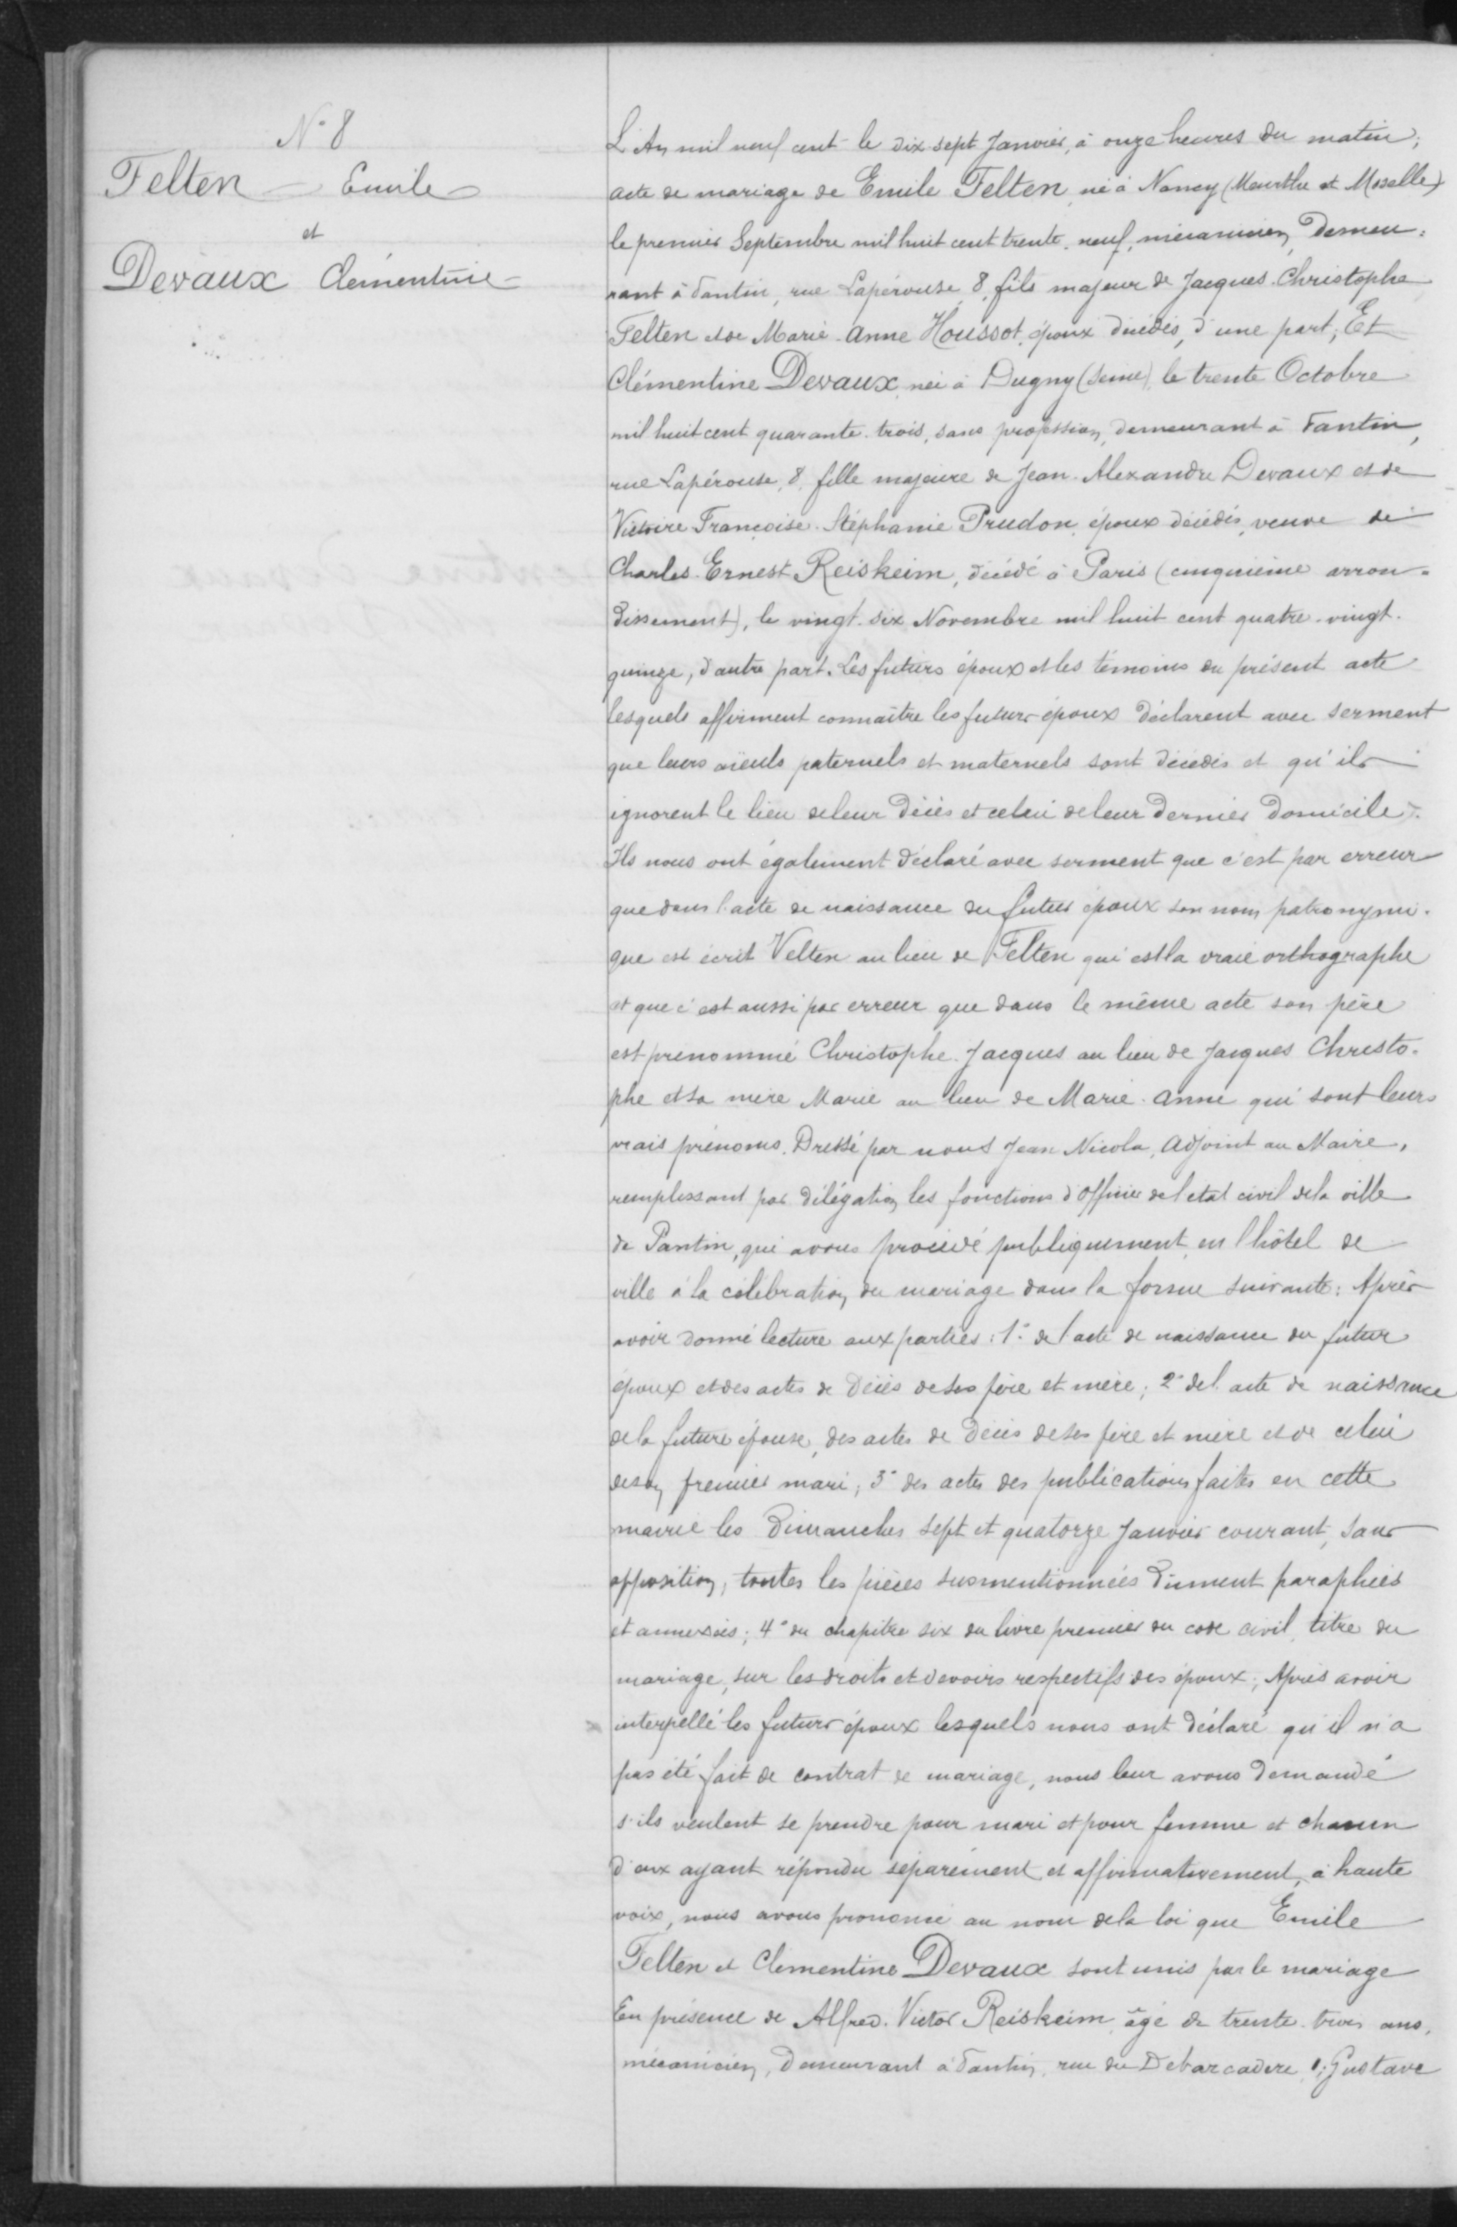N°8
Felten Emile
et
Devaux Clémentine
L'an mil neuf cent le dix sept Janvier, à onze heures du matin,
acte de mariage de Emile Felton, né à Nancy (Meurthe et Moselle)
le premier Septembre mil huit cent trente neuf, mécanicien, demeur
rant à Patin, rue Laperouse 8, fils majeur de Jacques Christophe
Felten et de Marie-Anne Houssot, époux décédés, d'une part, Et
Clémentine Devaux, née à Dugny (Seine) le trente Octobre
mil huit cent quarante trois sans profession, demeurant à Fantin
rue Lapérouse, 8, fille majeure de Jean-Alexandre Devaux et de
Victoire Françoise Stéphanie Prudon époux décédés, veuve de
Charles Ernest Reiskeim, décédé à Paris (cinquième arron
dissement) le vingt-six Novembre mil huit cent quatre-vingt
quinze, d'autre part. Les futurs époux et les témoins du présent acte
lesquels affirment connaître les futurs époux déclarent avec serment
que leurs aïeuls paternels et maternels sont décédés et qu'ils
ignorent le lieu deleur décès et celui de leur dernier domicili.
Ils nous ont également déclaré avec serment que c'est par erreur
que dans l'acte de naissance du futur époux son nom patronym
que est écrit Velten au lieu de Felten qui est la vraie orthographe
et que c'est aussi par erreur que dans le même acte son père
est prénommé Christophe Jacques au lieu de Jacque Christo-
phe etsa mère Marie au lieu de Marie-anne qui sont leurs
vrais prénoms. Dressé par nous Jean Nicola, Adjoint au Maire,
remplissant par délégation les fonctions d'Officier de l etat civil dela ville
de Pantin, qui avons procédé publiquement en l'hôtel de
ville à la célébration, du mariage dans la forme suivante: Après
avoir donné lecture aux parties: 1° de l'acte de naissance du futur
epoux et des actes de décès de ses père et mère; 2° de l'acte de naissance
de la futur épouse, des actes de décès deses père et mère et de celui
deson premier mari; 3° des actes des publications faites en cette
mairie les dimanches sept et quatorze Janvier courant, sans
oppositio, toutes les pièces susmentionnés dûment paraphées
et annexées; 4° du chapitre six du libre premier du code civil titre du
mariage, sur les droits et devoirs respectifs des époux, Après avoir
interpellé les futurs époux lesquels nous ont déclaré qu'il n'a
pas été fait de contrat de mariage, nous leur avons demandé
s'ils veulent se prendre pour mari et pour femme et chacun
d'eux ayant répondu séparément et affirmativement à haute
voix, nous avons prononcé au nom de la loi que Emile
Felten et Clementin Devaux sont unis par le mariage
En présence de Alfred Victore Reiskeim, âgé de trente-trois ans,
mécanicien, demeurant à Pantin, rue du Debarcadere Gustave
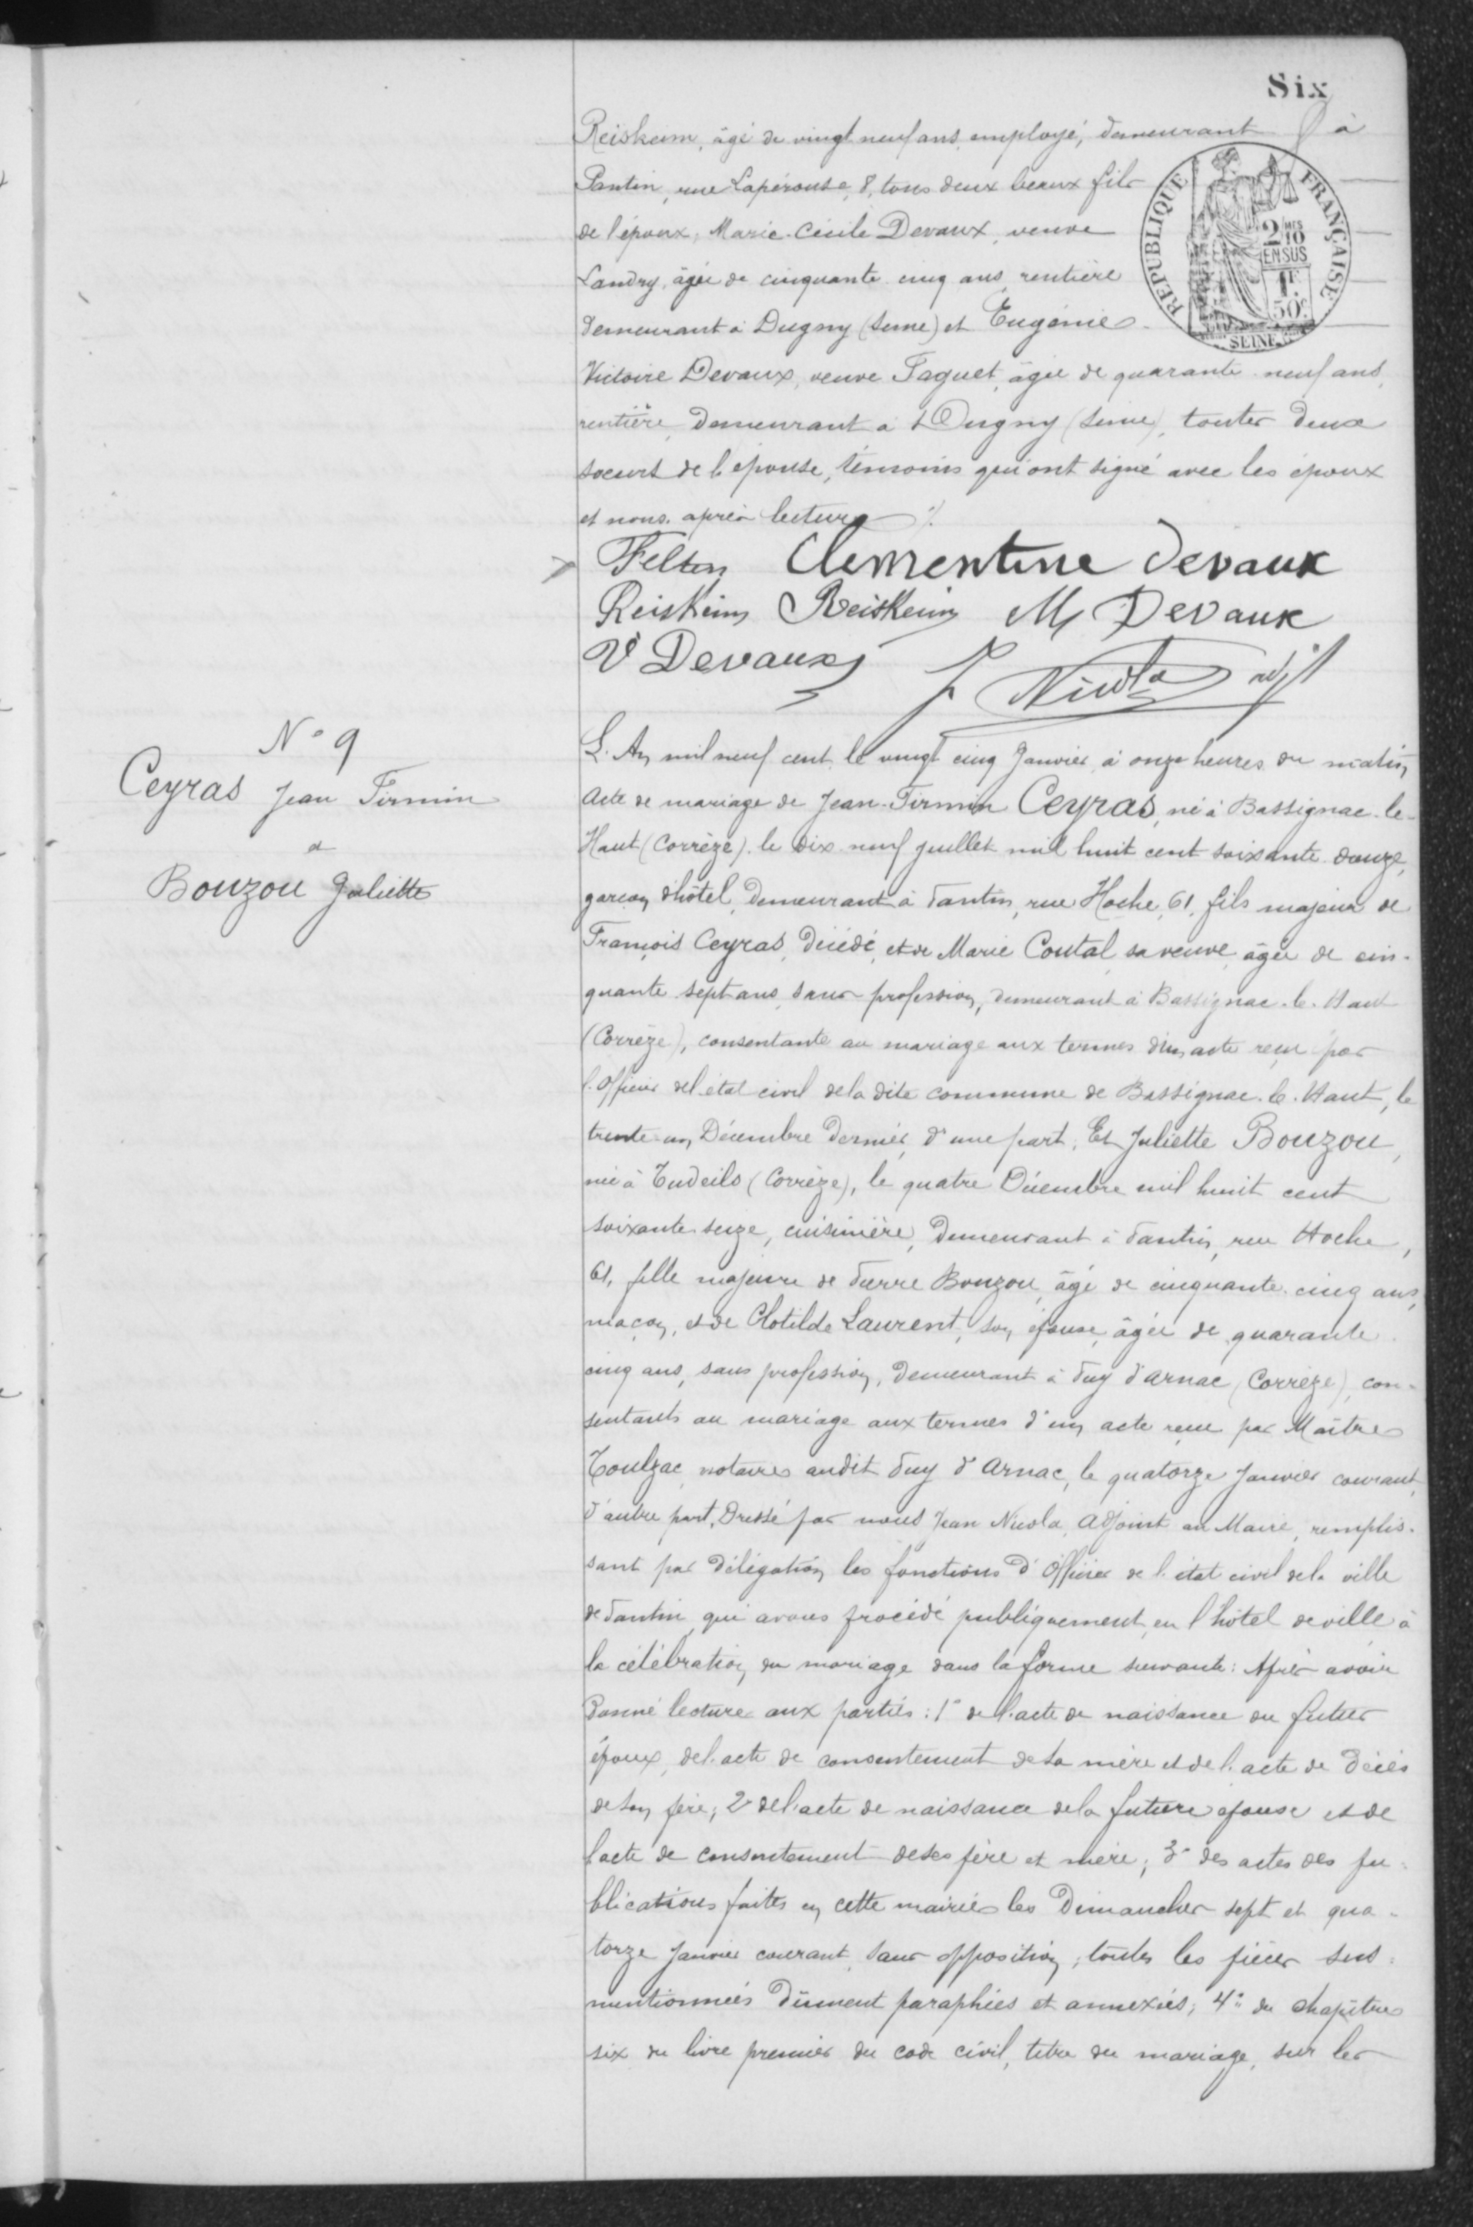Resikem, âgé de vingt neuf ans employé; demeurant à
Pantin, rue Lapérouse, 8, tous deux beaux fils
de l'époux; Marie-Cécile Devaux, veuve
Landry, âgée de cinquante cinq ans, rentière
demeurant à Dugny (Seine) et Eugénie
Victoire Devaux, veuve Faguet, âgée de quarante-neuf ans
rentière, demeurant à Dugny (Seine), toutes deux
soeurs de l'epouse, témoins qui ont signé avec les époux
et nous après lecture./.
N°9
Ceyras Jean Firmin
et
Bouzou Juliette
L'an mil neuf cent le vingt cinq Janvier à onze heures du matin
Acte de mariage de Jean-Firmin Ceyras, né à Bessignac-le-
Haut (Corrèze) le dix-neuf Juillet mil huit cent soixante douze,
garçon dhôtel, demeurant à Patin, rue Hoche, 61, fils majeur de
François Ceyras décédé et de Marie Coutal sa veuve âgé de cin-
quante sept ans sans profession, demeurant à Bassignac-le-Haut
(Corrèze), consentante au mariage aux termes d'un acte reçu par
l'Officier del état civil dela dite commune de Bessignac-le-Haut, le
trente-un Décembre dernier, d'une part. Et Juliette Bouzou,
né à Tudeuils (Corrèze), le quatre Décembre mil huit cent
soixante seize, cuisinière, demeurant à Pantin, rue Hoche,
61, fille majeure de Pierre Bonzou, âgé de cinquante-cinq ans,
maçon, et de Clotilde Laurent, son épouse, âgée de quarante
cinq ans, sans profession, demeurant à Puy d'arnac (Corrèze) con-
sentants au mariage aux termes d'un acte reçu par Maitre
Toulzac, notaire audit Puy d'Arnac, le quatorze Janvier courant
d'autre part. Dressé par nous Jean Nicola, Adjoint au Maire remplis-
sant par délégation les fonctions d'Officier de l'état civil dela ville
de Pantin qui avons procédé publiquement en l'hôtel de ville à
la célébration, du mariage dans la frome suivant: Après avoir
donné lecture aux parties: 1° de l'acte de naissance du futur
époux, del'acte de consentement de la mère et de l'acte de décès
de son père; 2° del'acte de naissance dela future épouse et de
l'acte de consentement de ses père et mère; 3° des actes pu
blications faites en cette mairie les dimanches sept et qua-
torze Janvier courant sans opposition, toutes les pièces sus
mentionnées dûment paraphées et annexées; 4° du chapitre
six du livre premier du code civil, titre du mariage sur les